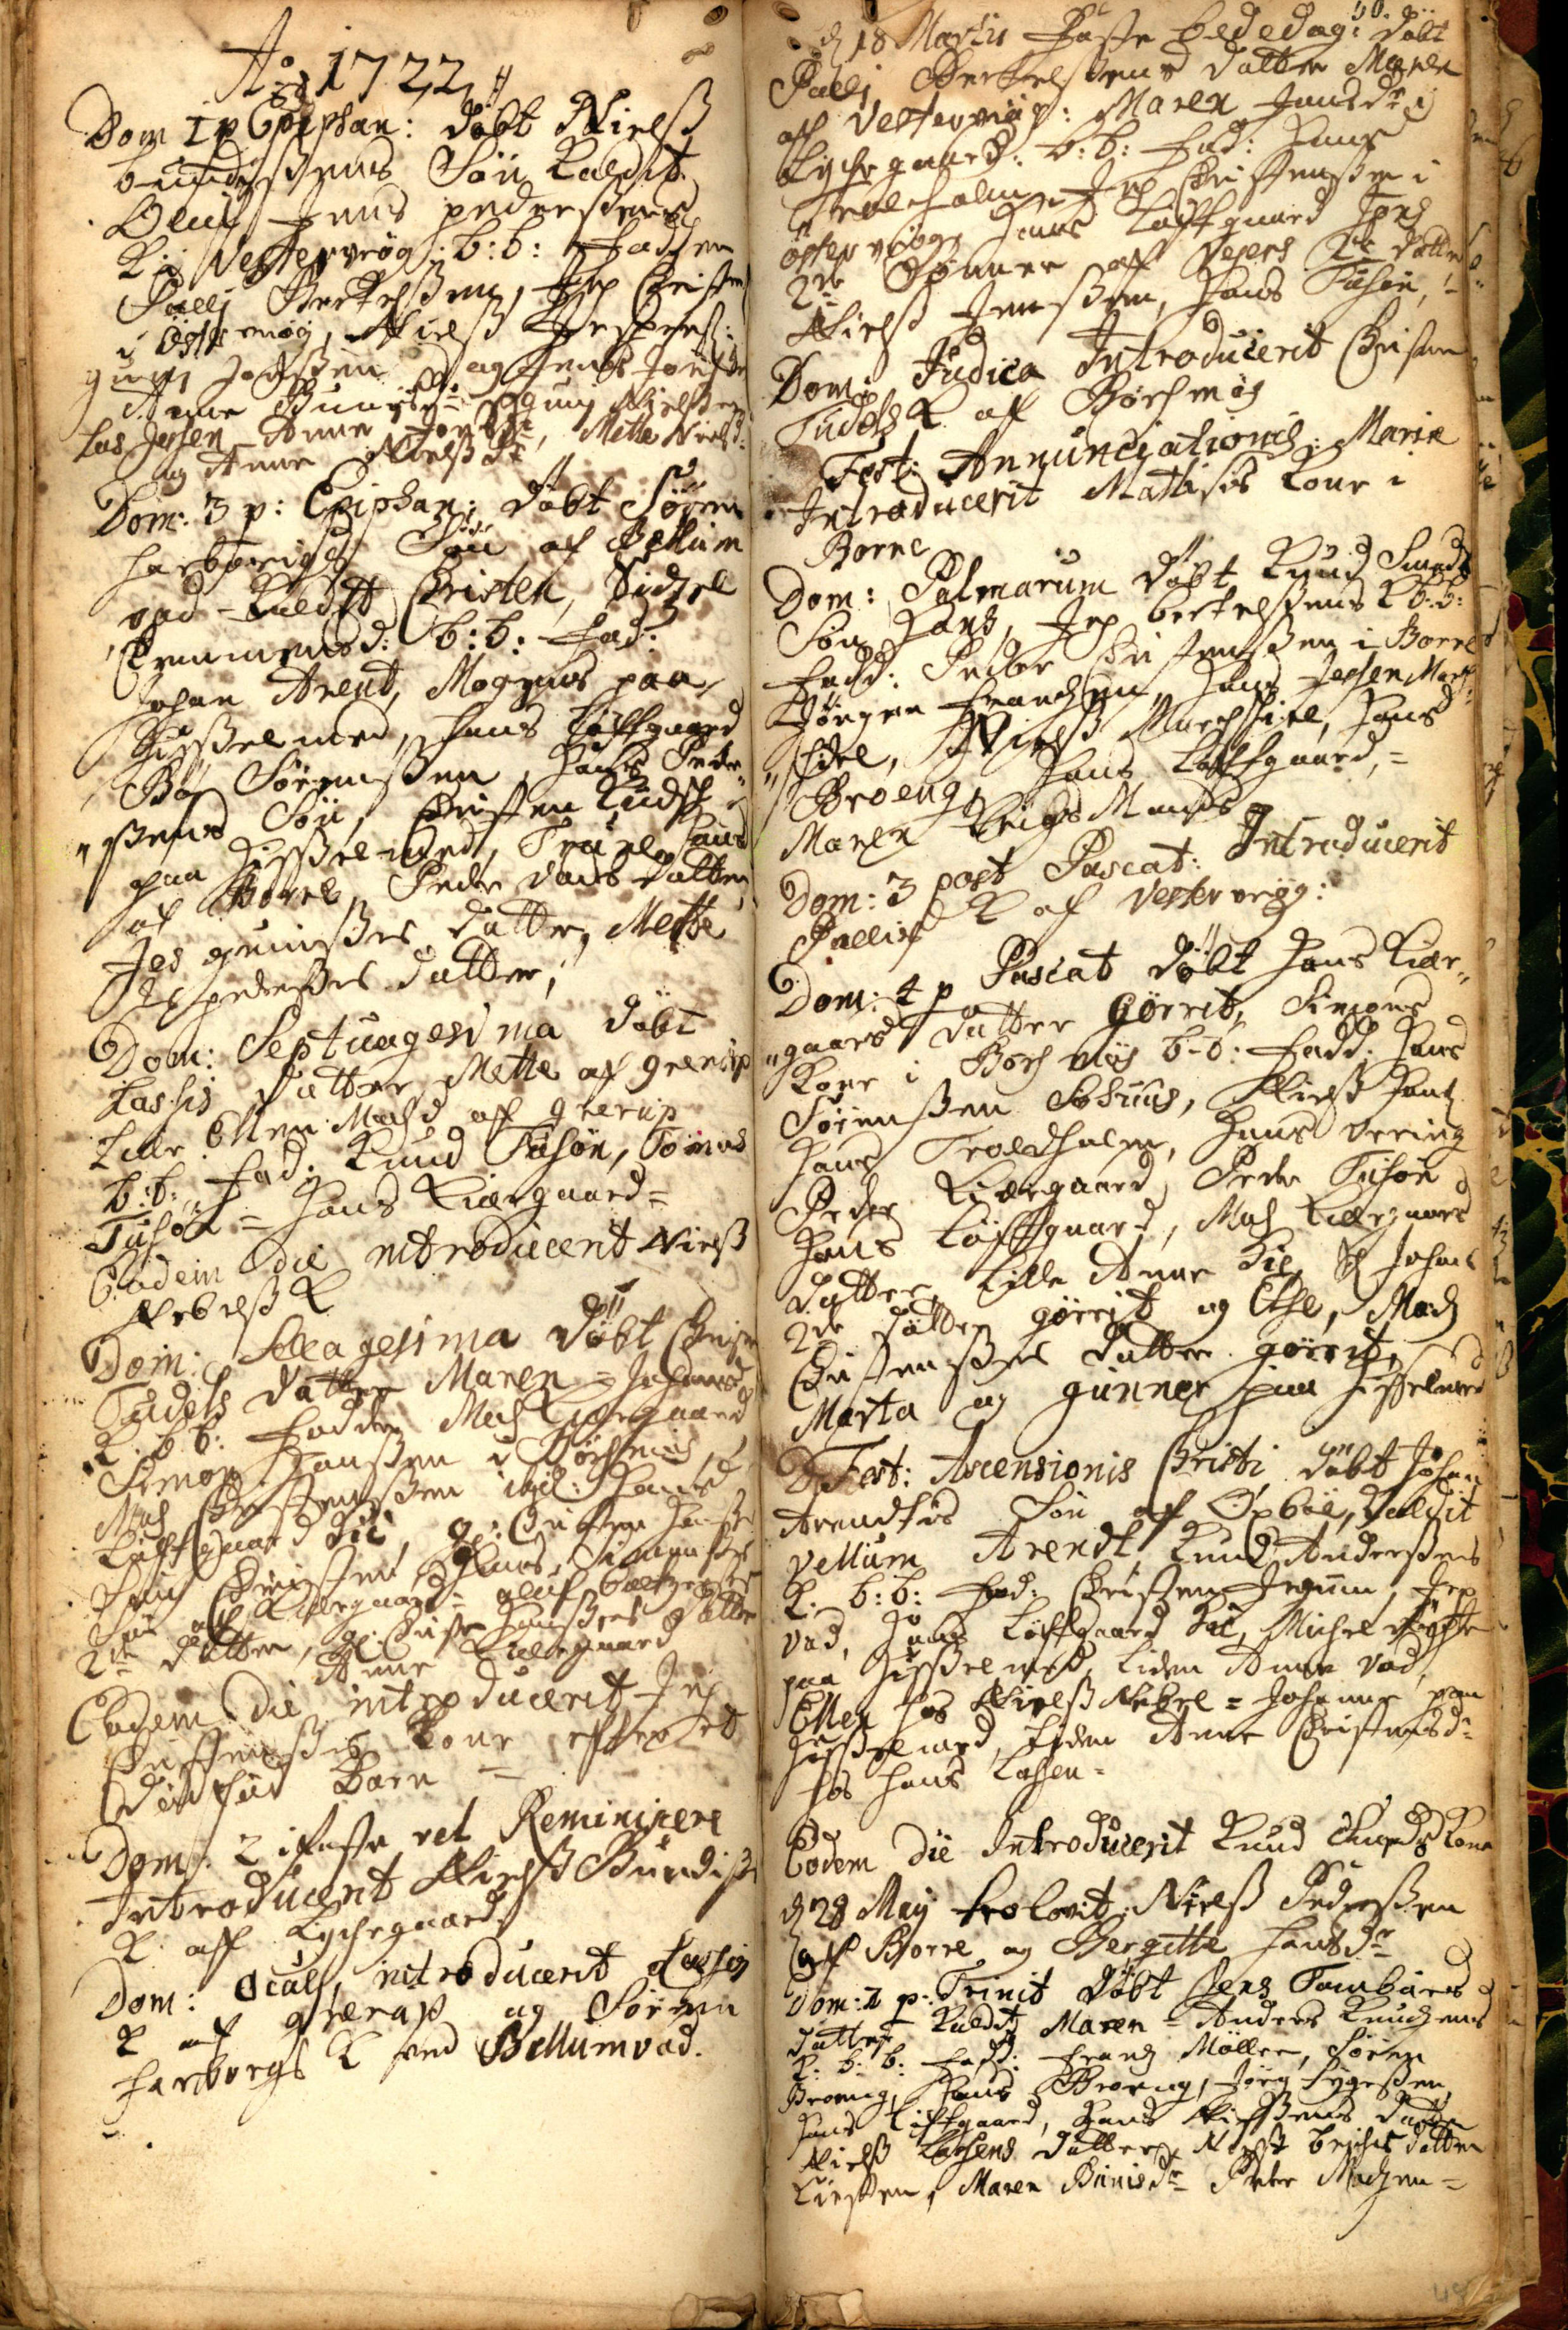Ao 1722 ##
Dom1 p Epiphan: døbt Niels
Bundisens Søn kaldit
Oluf Jens Pedersens
K i Vestervrøg: b:b: Faddere
Pallj Bertelsen, Jep Christensen
i Østervrøg, Niels Jespers:
Guni Jonsen og Jens Jonsen
Anne Bunisdr Gunj Nielsen
Las Jessen Anne Jonsdr, Mette Nielsd
og Anne Nielsdr
Dom: 3 p. Epiphan: døbt Søren
Harborigs Søn af Bellum
vad kaldit Christen, Sidzel
Clemmensd: b:b: Fad:
Johan Arent, Mogens paa
Hisselmed, Hans Løftgaard
Bøj Sørensen Hans Peder¬
sens Søn, Christen Kudsch
paa Hisselmed, Trine Hansd
af Borre, Peder Vads Datter
Jes Gunisens Datter, Mette
Jes Pedersens Datter,
Dom: Septuagesima døbt
Lassis Datter Mette af Grerup
Lille Ellen Madzd af Grerup
b:b: Fad: Knud Tusøn, Tomas
Tusøn= Hans Kiærgaard=
Eodem Die introducerit Niels
Nebels K
Dom: Sexagesima døbt Christen
Tudels Datter Maren= Johans
K. bb: Faddere Madz Kiærgaard
Simon Hansen i Børsmøs
Madz Christensen ibid: Hans
Løftgaard Ki##, gl: Christen Hansens
Søn Christen, Hans Simonsens
Søn af Kiærgaard= Oluf Baltzersens
2de Døttre, gl. Christen Hansens Datter
Anne Kiærgaard
Eodem Die introducerit Jep
Christensens Kone efter et
dødfød Barn
Dom: 2 i Faste vel Reminiscere
Introducerit Niels Bundis
K: af Lychegaard
Dom: Oculi introducerit Lassis
K af Grerup og Søren
Harborgs K ved Bellumvad
50
D 18 Martii Faste Bededag: døbt
Pallj Bertels Datter Maren
af Vestervrøg: Maren Jonsdr i
Lychegaard: b:b: Fad: Hans
Troldholm Jep Christensen i
Østervrøg, Hans Løftgaard Jons
2de Sønner af Vejers 2de Datter
Niels Jensen, Hans Tusøn
Dom: Judica Introducerit Christen
Tudels K af Børsmøs
Fest: Annunciationis: Mariæ
Introducerit Mattisis Kone i
Borre
Dom: Palmarum døbt Knud Smeds
Søn Hans, Jep Bertelsens K b:b:
Fadd: Peder Christensen i Borre
Jørgen Frandzen Hans Jessen Marsch¬
schiel, Niels Marchschiel, Hans
Broeng, Hans Løftgaard,=
Maren Krigs Mands
Dom: 3 post Pascat: Introducerit
Pallis K af Vestervrøg:
Dom: 4 p Pascat døbt Hans Kiær¬
gaards Datter Gørret, Simons
Kone i Børsmøs b-b: Fadd: Hans
Sørensen Søhuus, Niels Hans
Hans Troldholm, Hans Vering
Peder Kiærgaard Peder Tusøn
Hans Løftgaard, Madz Kiærgaars
Datter, Lille Anne Kie##, Sl Johans
2de Døttre Gørrit og Else, Madz
Christensens Datter Gørrit,
Marta og Gunner paa Hisselmed
Fest: Ascensionis Christi døbt Johan
Arendtis Søn af Oxbøl, kaldit
Vellum Arendt, Knud Andersens
K. b:b: Fad: Christen Jegum, Jep
Vad, Hans Løftgaard Kil##, Michel Fogde
paa Hisselmed, Liden Anne Vad,
Ellen hos Niels Nebel=  Johanne paa
Hisselmed, Liden Anne Christensdr
hos Hans Lassen=
Eodem Die Introducerit Knud Smeds Kone
D 28 Maij trolovit: Niels Pedersen
af Borre og Bergitte Hansdr
Dom: 2 p: Trinit døbt Jens Tamburs
Datter kaldet Maren= Anders Knudzens
K: b:b: Fadd: Frandz Møller, Søren
Broeng, Hans Broeng, Jørg Tygesen
Hans Liftgaard, Hans Nissens Datter
Niels Lassens Datter, Niels Brichis Datter
Kirsten, Maren Bunisdr Peder Madzen=